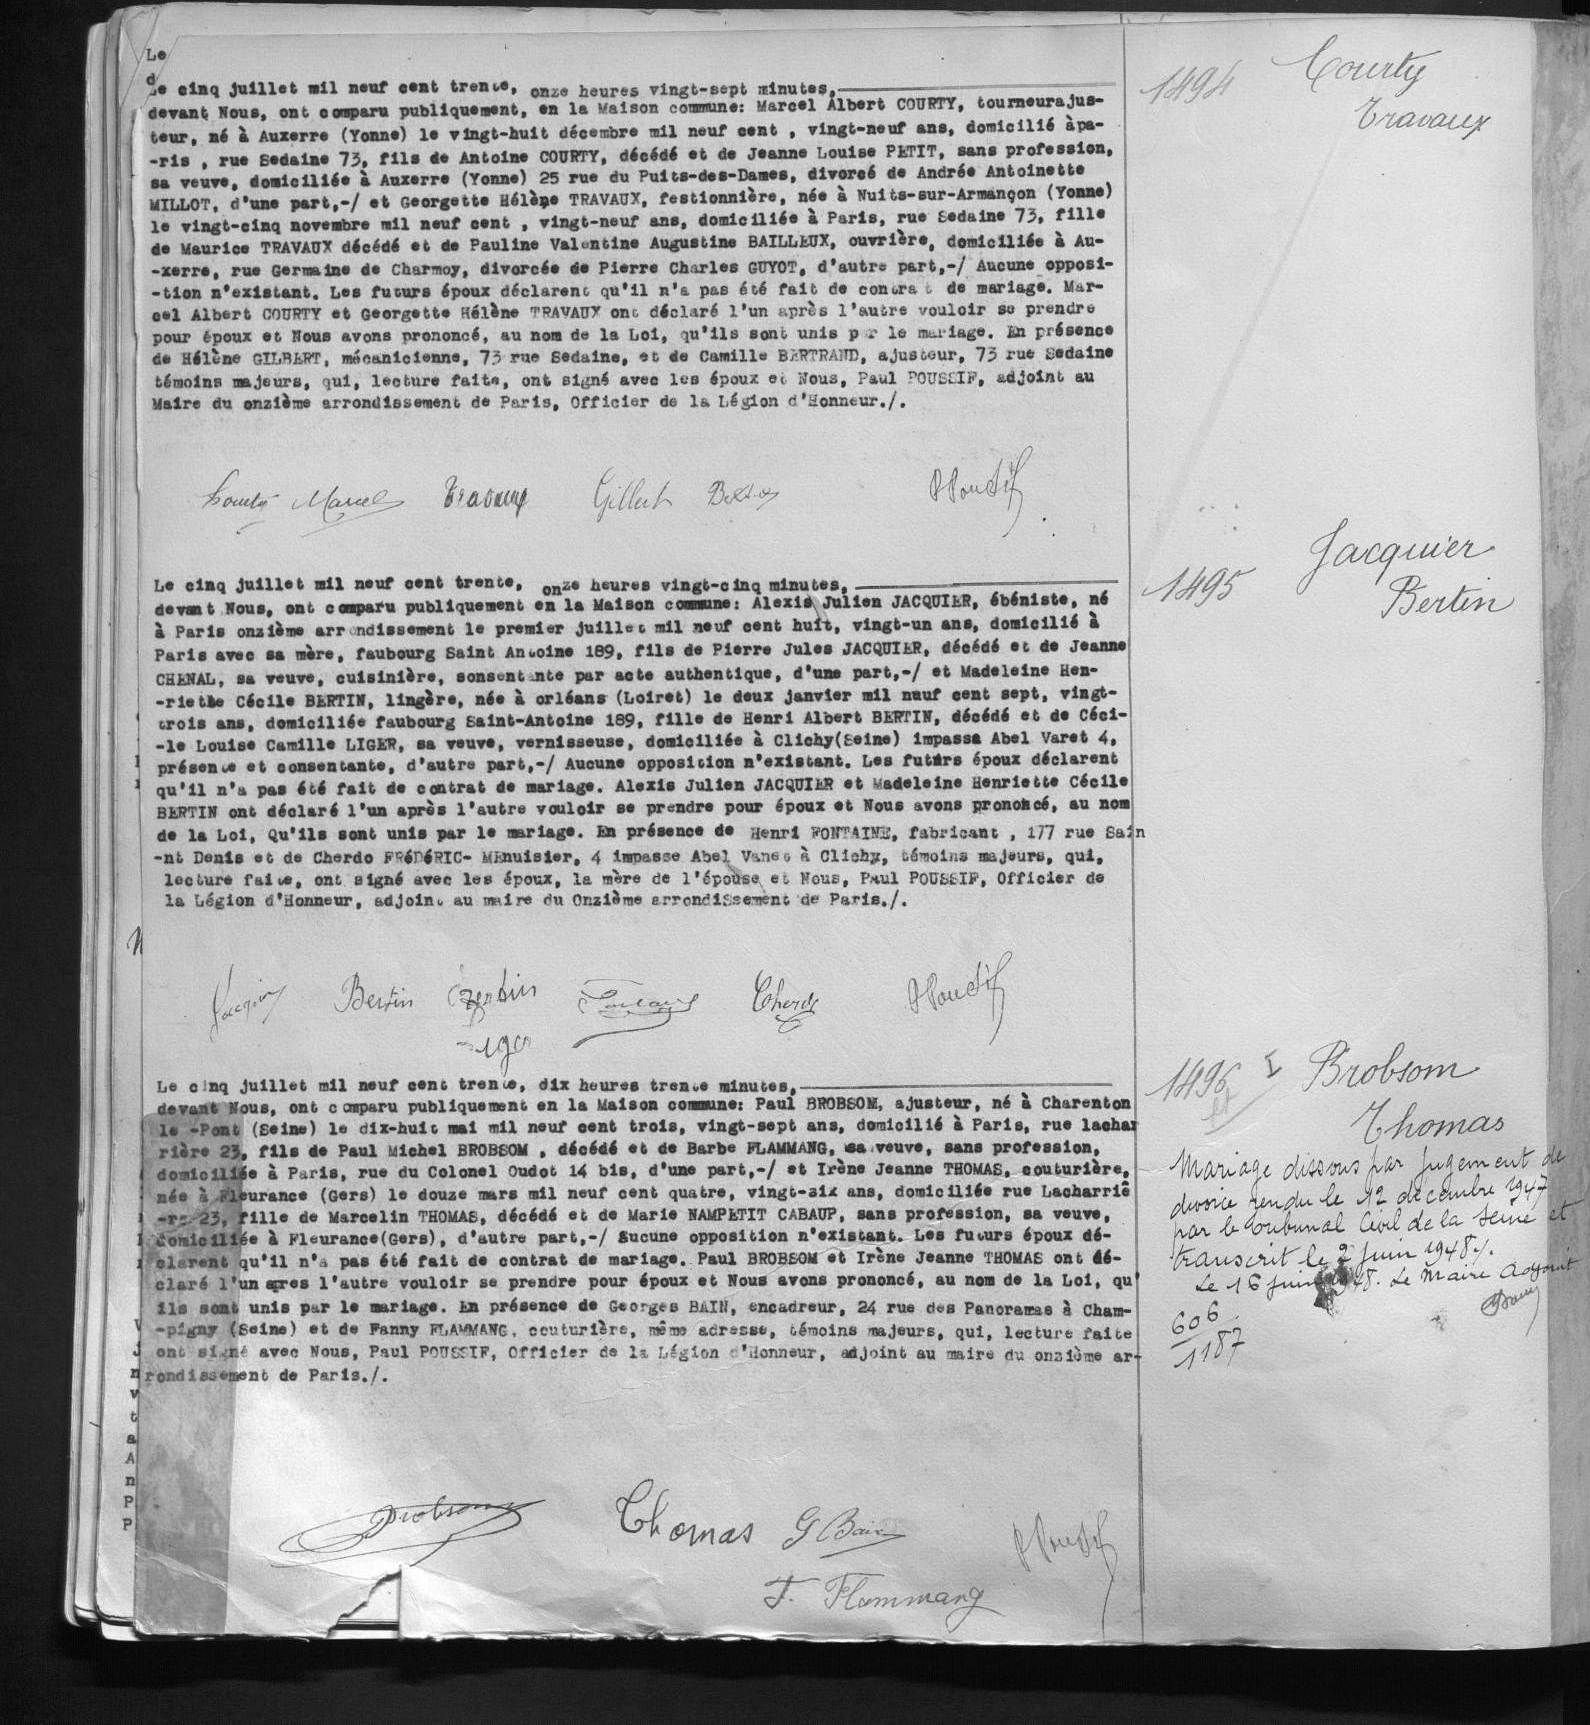Le cinq juillet mil neuf cent trente, onze heures vingt-sept minutes ,-
devant Nous, ont comparu publiquement, en la Maison commune: Marcel Albert COURTY, tourneur a jus-
teur, né à Auxerre (Yonne) le vingt-huit décembre mil neuf cent, vingt-neuf ans, domicilié à pa-
-ris, rue Sedaine 73, fils de Antoine COURTY, décédé et de Jeanne Louise PETIT, sans profession,
sa veuve, domiciliée à Auxerre (Yonne) 25 rue du Puits-des-Dames, divorcé de Andrée Antoinette
MILLOT, d'une part ,-/ et Georgette Hélène TRAVAUX, festionnère, née à Nuits-sur-Armançon (Yonne)
le vingt-cinq novembre mil neuf cent, vingt-neuf ans, domiciliée à Paris, rue Sedaine 73, fille
de Maurice TRAVAUX décédé et de Pauline Valentine Augustine BAILLEUX, ouvrière, domicilié à Au-
-xerre, rue Germaine de Charmoy, divorcée de Pierre Charles GUYOT, d'autre part ,-/ Aucune opposi-
-tion n'existant. Les futurs époux déclarent qu'il n'a pas été fait de contra de mariage. Mar-
cel Albert COURTY et Georgette Hélène TRAVAUX ont délcaré l'un après l'autre vouloir se prendre
pour époux et Nous avons prononcé, au nom de la Loi, qu'ils sont unis par le mariage. En présence
de Hélène GILBERT, mécanicienne, 73 rue Sedaine, et de Camille BERTRAND, ajusteur, 73 rue Sedaine
témoins majeurs, qui, lecture faite, ont signé avec les époux et Nous, Paul POUSSIF, adjoint au
Maire du onzième arrondissement de Paris. Officier de la Légion d'Honneur ./.
Le cinq juillet mil neuf cent trente, onze heures vingt-cinq minutes, -
devant Nous, ont comparu publiquement en la Maison commune: Alexis Julien JACQUIER, ébéniste, né
à Paris, onzième arrondissement le premier juillet mil neuf cent huit, vingt-un ans, domicilié à
Paris avec sa mère, faubourg Saint Antoine 189, fils de Pierre Jules JACQUIER, décédé et de Jeann
CHENAL, sa veuve, cuisinière, sonsentante par acte authentique, d'une part ,-/ et Madeleine Hen-
-riette Cécile BERTIN, lingène, née à orléans (Loiret) le deux janvier mil neuf cent sept, vingt-
trois ans, domiciliée faubourg Saint-Antoine 189, fille de Henri Albert BERTIN, décédé et de Céci)
-le Louise Camille LIGER, sa veuve, vernisseuse, domiciliée à Clichy (Seine) impassa Abel Varet 4,
présente et consentante, d'autre part ,-/ Aucune opposition n'existant. Les futurs époux déclarent
qu'il n'a pas été fait de contrat de mariage. Alexis Julien JACQUIER et Madeleine Henriette Cécile
BERTIN ont déclaré l'un après l'autre vouloir se prendre pour époux et Nous avons prononcé, au nom
de la Loi, Qu'ils sont unis par le mariage. En présence de Henri FONTAINE, fabricant, 177 rue Sain
-nt Denis et de Cherdo FREDERIC-MEnuisier, 4 impasse Abel Vanec à Clichy, témoins majeurs, qui,
lecture faite, ont signé avec les époux, la mère de l'épouse de Nous, Paul POUSSIF, Officier de
la Légion d'Honneur, adjoint au maire du Onzième arrondissement de Paris ./.
Le cinq juillet mil neuf cent trente, dix heures trente minutes, -
devant Nous, ont comparu publiquement en la Maison commune: Paul BROBSON, ajusteur, né à Charenton
le-Pont (Seine) le dix-huit mai mil neuf cent trois, vingt-sept ans, domicilié à Paris, rue lachar
rière 23, fils de Paul Michel BROBSOM, décédé et de Barbe FLAMMANG, sa veuve, sans profession,
domiciliée à Paris, rue du Colonel Oudot 14 bis, d'une part ,-/ et Irène Jeanne THOMAS, couturière,
née à Fleurance (Gers) le douze mars mil neuf cent quatre, vingt-six ans, domiciliée rue Lacharriê-
rs 23, fille de Marcelin THOMAS, décédé et de Marie NAMPETIT CABAUF, sans profession, sa veuve,
domiciliée à Fleurance (Gers), d'autre part ,-/ Aucune opposition n'existant. Les futurs époux dé-
clarent qu'il n'a pas été fait de contrat de mariage. Paul BROBSOM et Irène Jeanne THOMAS ont dé-
claré l'un apres l'autre vuoloir se prendre pour époux et Nous avons prononcé, au nom de la LOi, qu'
ils sont unis par le mariage. En présence de Georges BAIN, encadreur, 24 rue des Panoramas à Cham-
-pigny (Seine) et de Fanny FLAMMANG, couturière, même adresse, témoins majeurs, qui, lecture faite
ont signé avec Nous, Paul POUSSIF, Officier de la Légion d'Honneur, adjoint au maire du onzième ar-
ondissement de Paris ./.
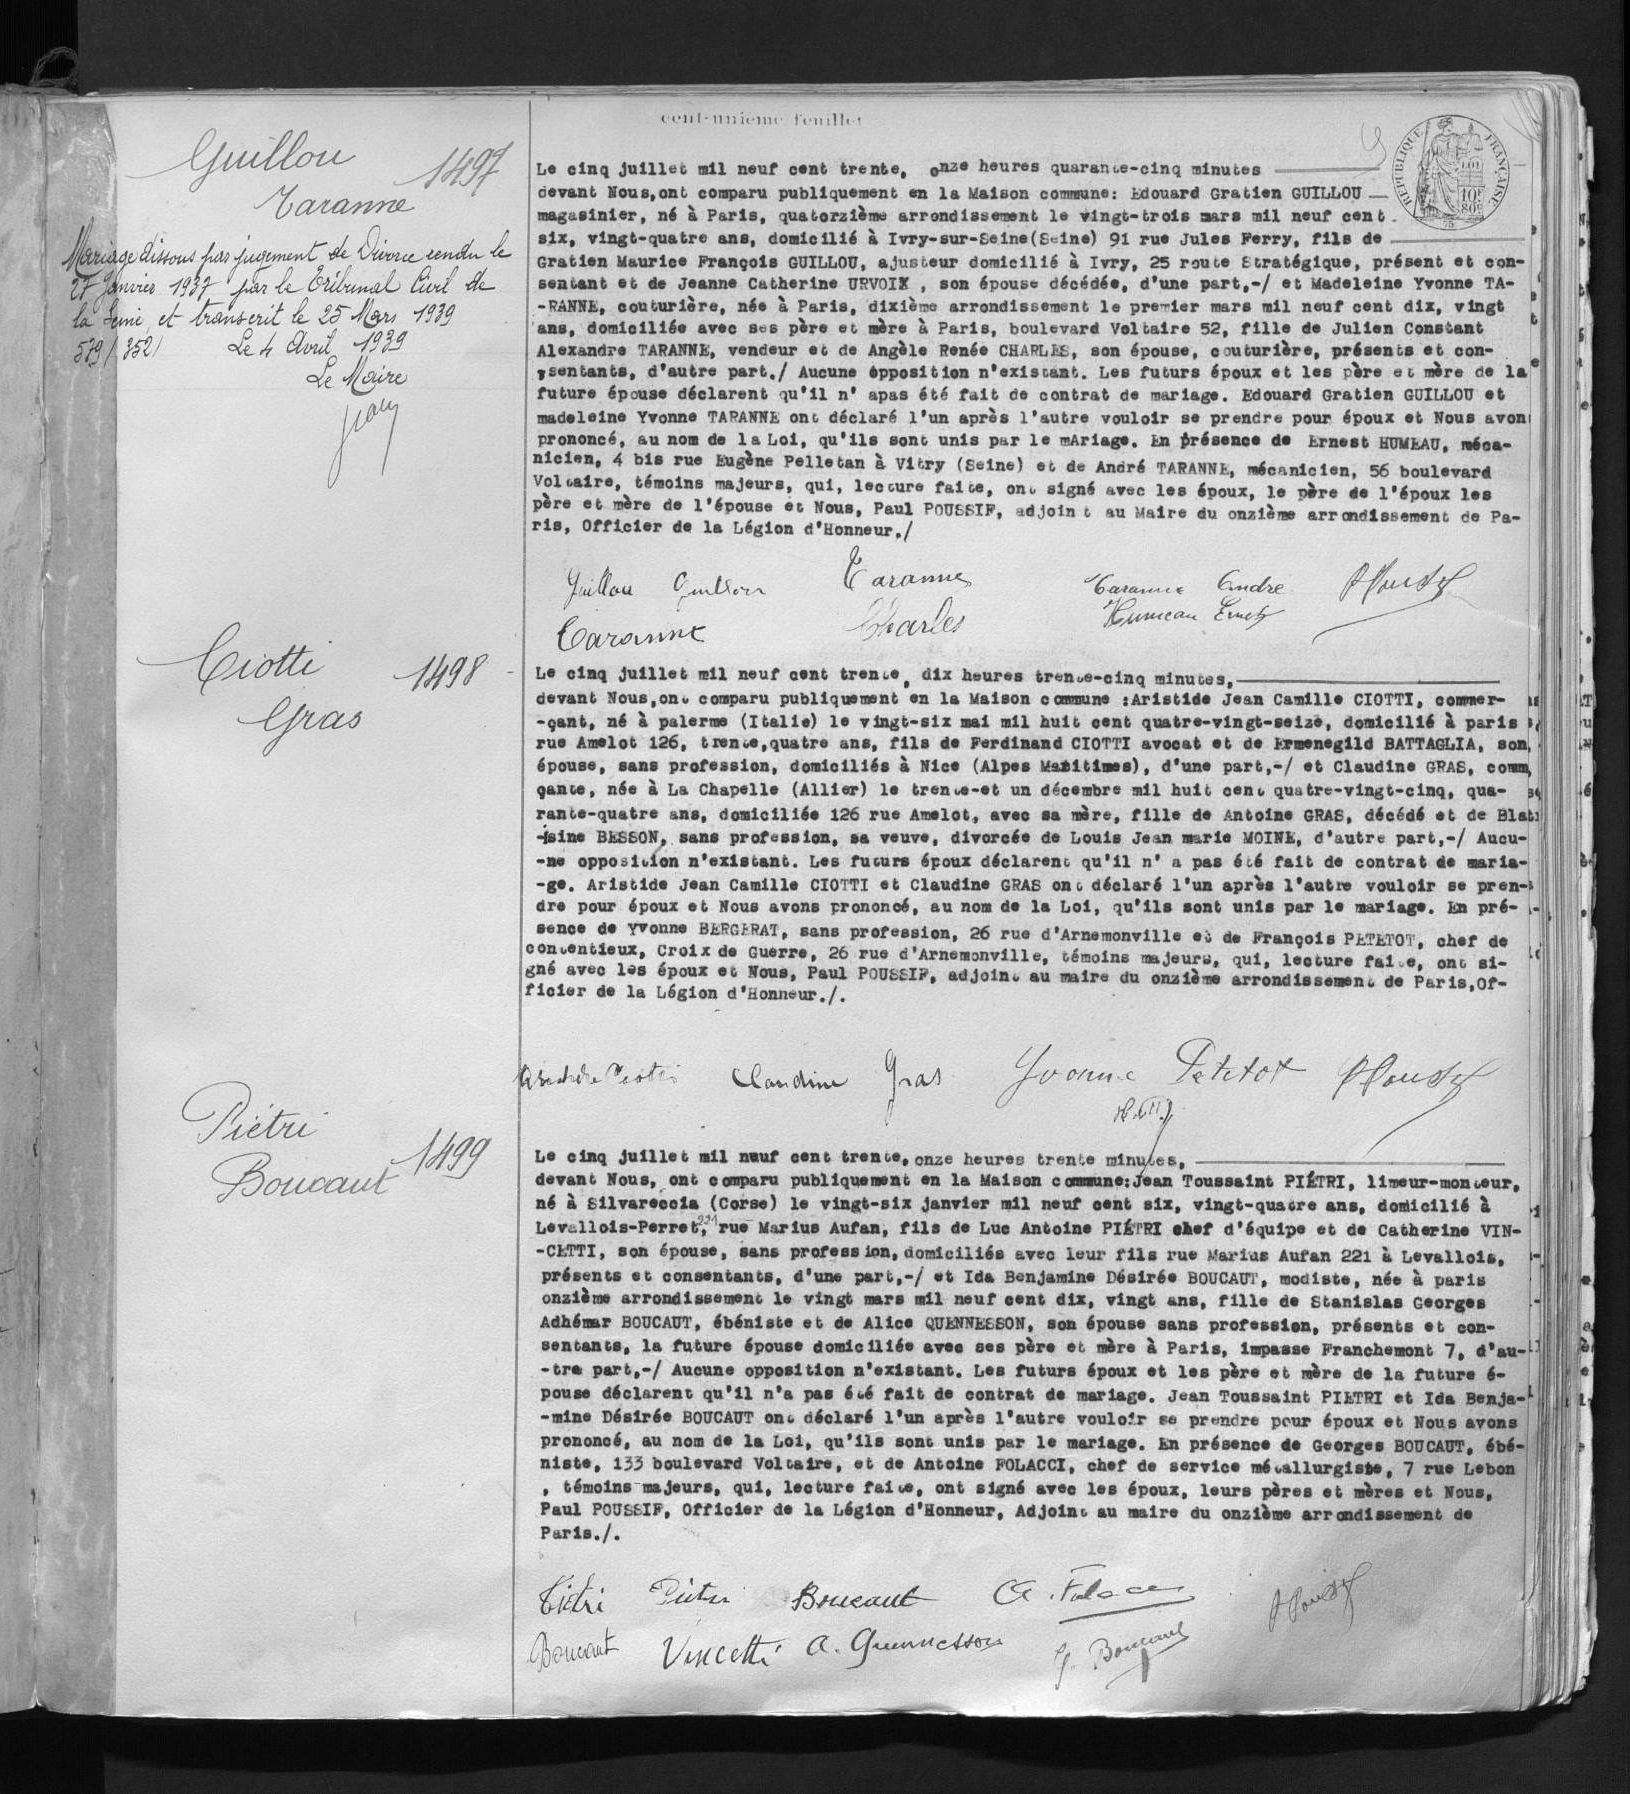Le cinq juillet mil neuf cent trente, onze heures quarante-cinq minutes -
devant Nous, ont comparu publiquement en la Maison commune: Edouard Gratien GUILLOU -
magasinier, né à Paris, quatorzième arrondissement le vingt-trois mars mil neuf cent
six, vingt-quatre ans, domicilié à Ivry-sur-Seine (Seine) 91 rue Jules Ferry, fils de -
Gratien Maurice François GUILLOU, ajusteur domicilié à Ivry, 25 route Stratégique, présent et con-
sentant et de Jeanne Catherine URVOIX, son épouse décédée, d'une part ,-/ et Madelein Yvonne TA-
-RANNE, couturière, née à Paris, dixième arrondissement le premier mars mil neuf cent dix, vingt
ans, domiciliée avec ses père et mère à Paris, boulevard Voltaire 52, fille de Julien Constant
Alexandre TARANNE, vendeur et de Angèle Renée CHARLES, son épouse, couturière, présents et con-
-sentants, d'autre part ./ Aucune opposition n'existant. Les futurs époux et les père et mère de la
future épouse déclarent qu'il n'a pas été fait de contrat de mariage. Edouard Gratien GUILLOU et
madeleine Yvonne TARANNE ont déclaré l'un après l'autre vouloir se prendre pour époux et Nous avon
prononcé, au nom de la Loi, qu'ils sont unis par le mAriage. En présence de Ernest HUMEAU, méca-
nicien, 4 bis rue Eugène Pelletan à Vitry (Seine) et de André TARANNE, mécanicien, 56 boulevard
Voltaire, témoins majeurs, qui, lecture faite, ont signé avec les époux, le père de l'époux les
père et mère de l'épouse et Nous, Paul POUSSIF, adjoint au Maire du onzième arrondissement de Pa-
ris, Officier de la Légion d'Honneur ./
Le cinq juillet mil neuf cent trente, dix heures trente-cinq minutes ,-
devant Nous, ont comparu publiquement en la Maison commune: Aristide Jean Camille CIOTTI, commer-
-çant, né à palerme (Italie) le vingt-six mai mil huit cent quatre-vingt-seize, domicilié à paris
rue Amelot 126, trente, quatre ans, fils de Ferdinand CIOTTI avocat et de Ermenegild BATTAGLIA, son
épouse, sans profession, domiciliés à Nice (Alpes Maritimes), d'une part ,-/ et Claudine GRAS, comm
cante, né à la Chapelle (Allier) le trente-et un décembre mil huit cent quatre-vingt-cinq, qua-
rante-quatre ans, domiciliée 126 rue Amelot, avec sa mère, fille de Antoine GRAS, décédé et de Bla
-isine BESSON, sans profession, sa veuve, divorcée de Louis Jean marie MOINE, d'autre part ,-/ Aucu-
-ne opposition n'existant. Les futurs époux déclarent qu'il n'a pas été fait de contrat de maria-
-ge. Aristide Jean Camille CIOTTI et Claudine GRAS ont déclaré l'un après l'autre vouloir se pren-
dre pour époux et Nous avons prononcé, au nom de la Loi, qu'ils sont unis par le mariage. En pré-
sence de Yvonne BERGERAT, sans profession, 26 rue d'Arnemonville et de François PETETOT, chef de
contentieux, Croix de Guerre, 26 rue d'Arnemonville, témoins majeurs, qui, lecture faite, ont si-
gné avec les époux et Nous, Paul POUSSIF, adjoint au maire du onzième arrondissement de Paris, Of-
ficier de la Légion d'Honneur ./.
Le cinq juillet mil neuf cent trente, onze heures trente minutes ,-
devant Nous, ont comparu publiquement en la Maison commune: Jean Toussaint PIETRI, limeur-monteur,
né à Silvareccia (Corse) le vingt-six janvier mil neuf cent six, vingt-quatre ans, domicilié à
Levallois-Perret, 221 rue Marius Aufan, fils de Luc Antoine PIETRI chef d'équipe et de Catherine VIN-
-CETTI, son épouse, sans profession, domiciliés avec leur fils rue Marius Aufan 221 à Levallois,
présents et consentants, d'une part ,-/ et Ida Benjamine Désirée BOUCAUT, modiste, née à paris
onzième arrondissement le vingt mars mil neuf cent dix, vingt ans, fille de Stanislas Georges
Adhémar BOUCAUT, ébeniste et de Alice QUENNESSON, son épouse, sans profession, présents et con-
sentants, la futurs épouse domiciliée avec ses père et mère à Paris, impasse Franchemont 7, d'au-
-tre part ,-/ Aucune opposition n'existant. Les futurs époux et les père et mère de la future é-
pouse déclarent qu'il n'a pas été fait de contrat de mariage. Jean Toussaint PIETRI et Ida Benja-
-mine Désiré BOUCAUT ont déclaré l'un après l'autre vouloir se prendre pour époux et Nous avons
prononcé, au nom de la Loi, qu'ils sont unis par le mariage. En présence de Georges BOUCAUT, ébé-
niste, 113 boulevard Voltaire, et de Antoine FOLACCI, chef de service mécallurgiste, 7 rue Lebon
, témoins majeurs, qui, lecture faite, ont signé avec les époux, leurs pères et mères et Nous,
Paul POUSSIF, Officier de la Légion d'Honneur, Adjoint au maire du onzième arrondissement de
Paris ./.